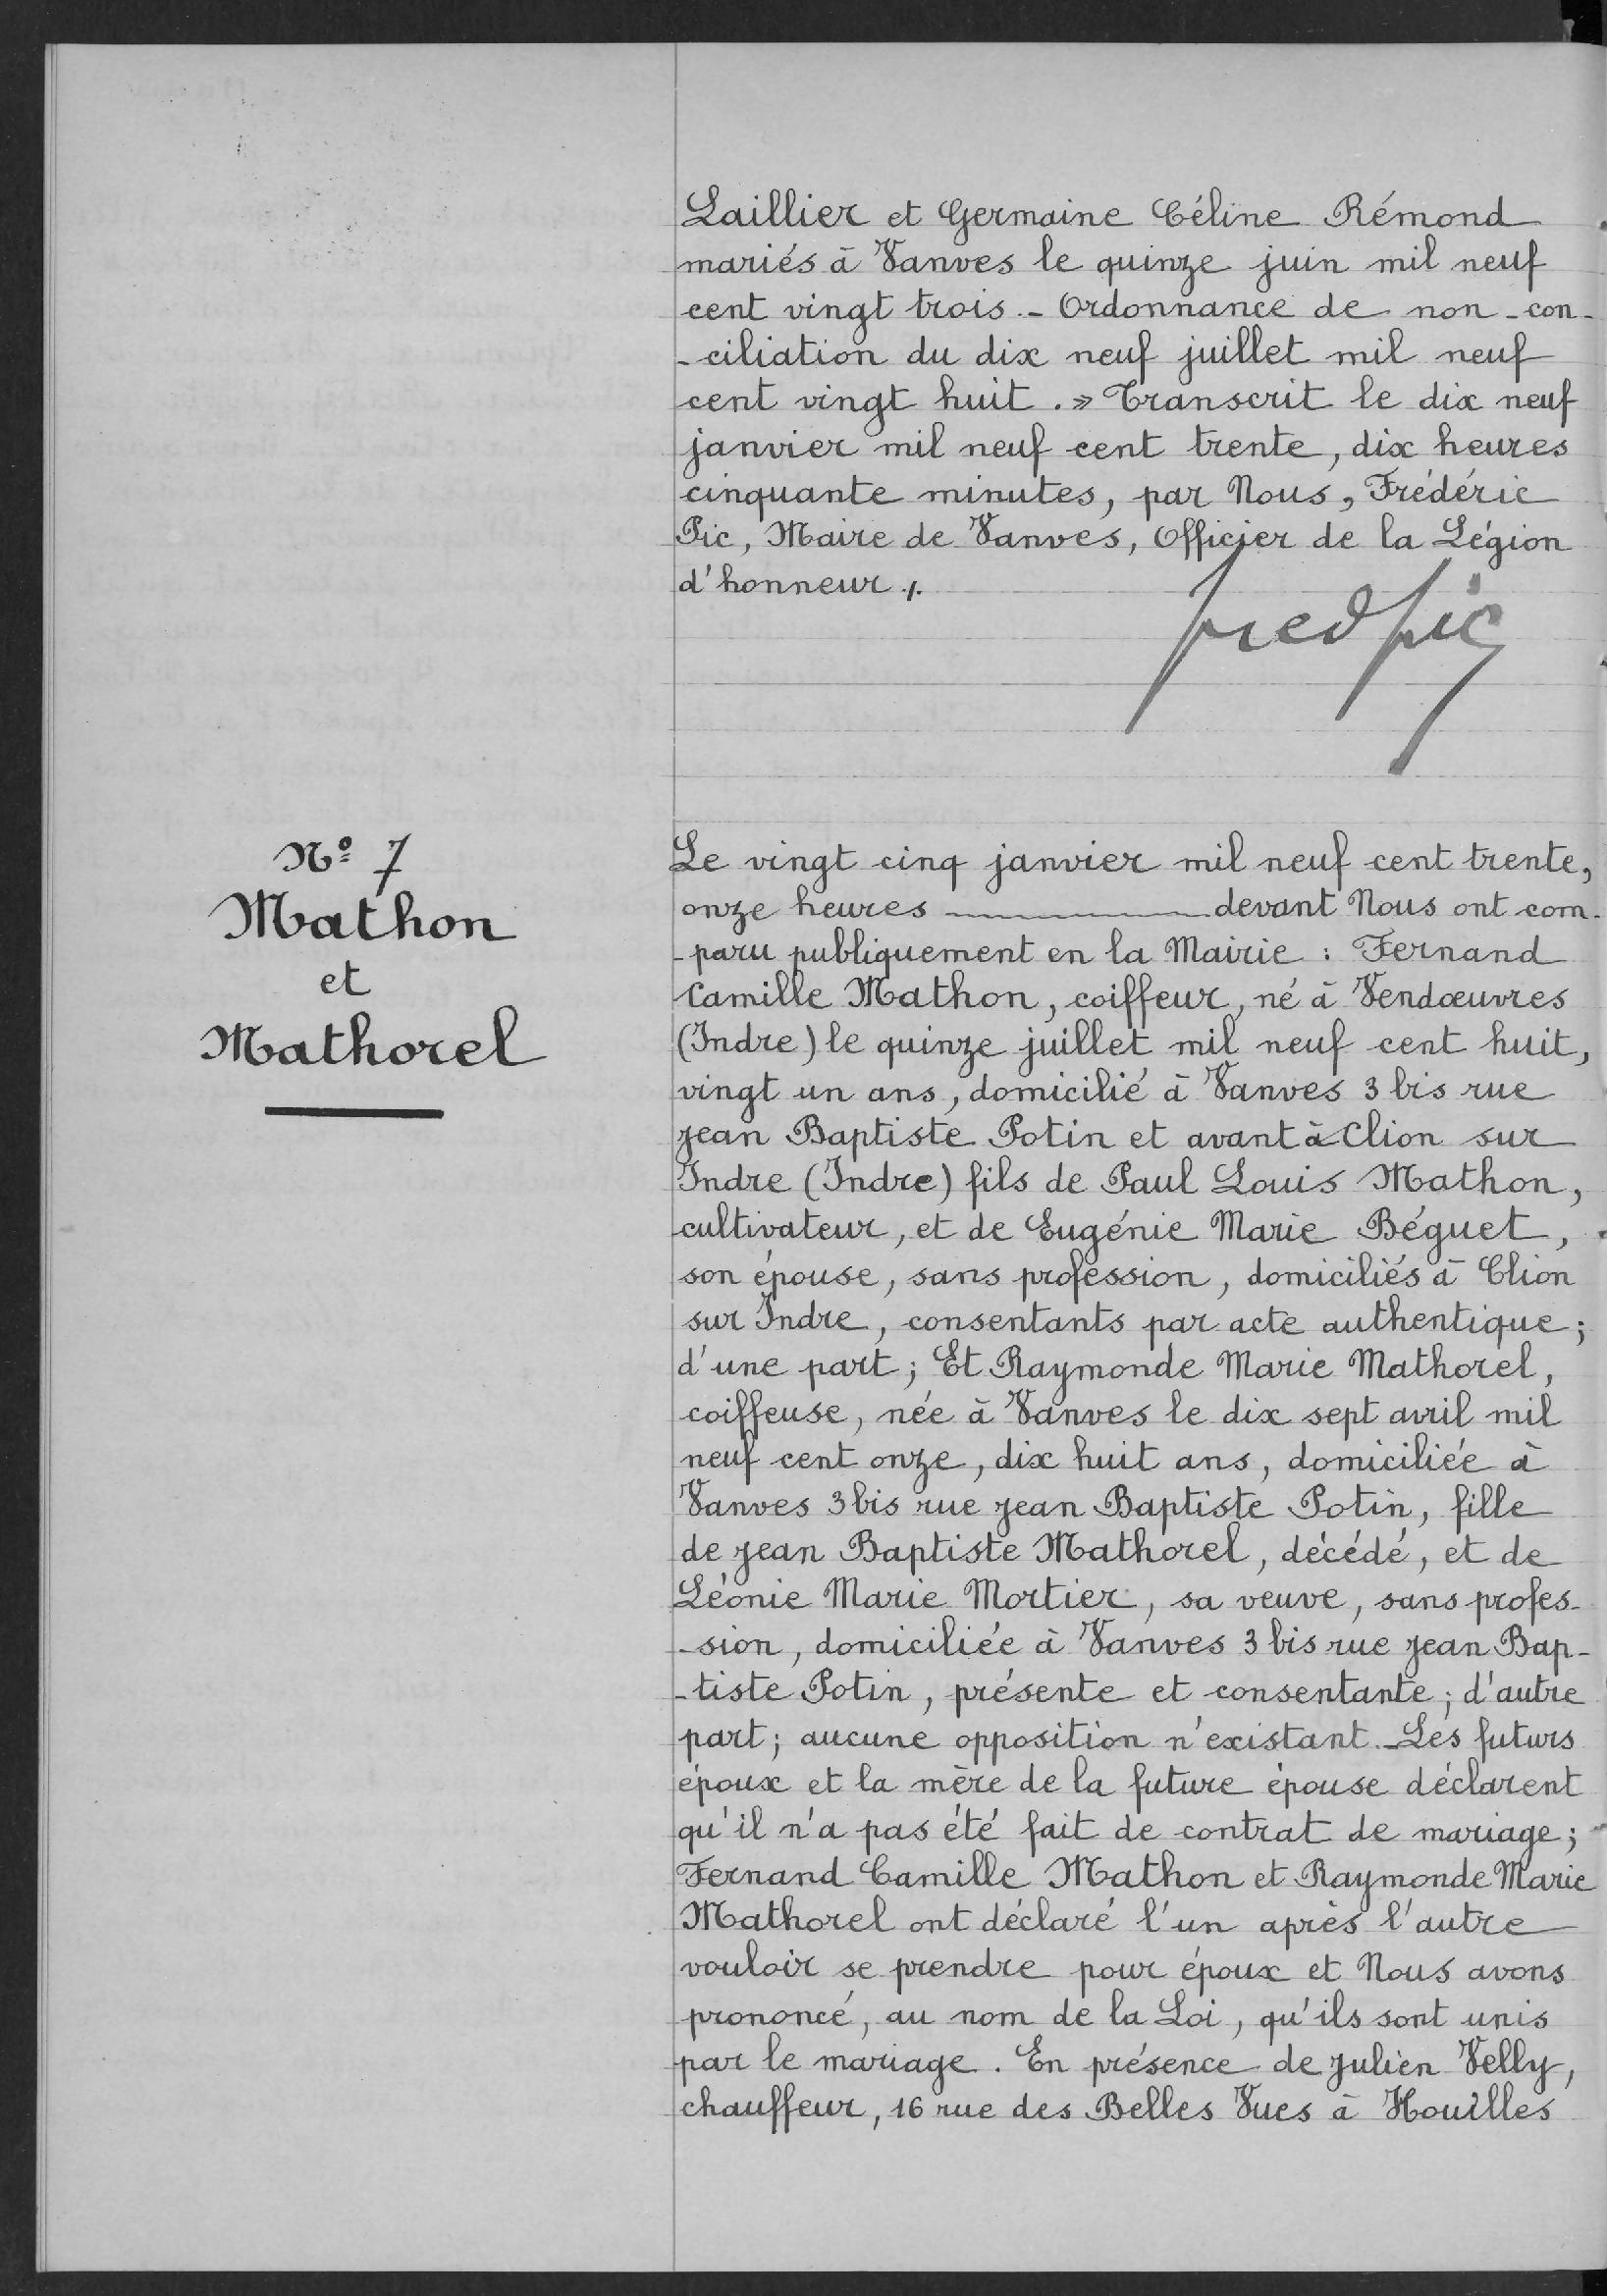Laillier et Germaine Céline Rémond
mariés à Vanves le quinze juin mil neuf
cent vingt trois.-Ordonnance de non-con-
-ciliation du dix neuf juillet mil neuf
cent vingt huit." Transcrit le dix neuf
janvier mil neuf cent trente, dix heures
cinquante minutes, par Nous, Frédéric
Pic, Maire de Vanves, Officier de la Légion
d'honneur./.
N°7
Mathon
et
Mathorel
Le vingt cinq janvier mil neuf cent trente
onze heures -devant Nous ont com-
-paru publiquement en la Mairie: Fernand
Camille Mathon, coiffeur, né à Vendoeuvres
(Indre) le quinze juillet mil neuf cent huit
vingt un ans, domicilié à Vanves 3bis rue
Jean Baptiste Potin et avant à Clion sur
Indre (Indre) fils de Paul Loui Mathon,
cultivatuer, et de Eugénie Marie Béguet,
son épouse, sans profession, domiciliés à Clion
sur Indre, consentants par acte authentique;
d'une part; Et Raymonde Marie Mathorel,
coiffeuse; née à Vanves le dix sept avril mil
neuf cent onze, dix huit ans, domiciliée à
Vanves 3 bis rue Jean Baptiste Potin, fille
de Jean Baptiste Mathorel, décédé et de
Léonie Marie Mortier, sa veuve, sans profes-
-sion, domiciliée à Vanves 3 bis rue Jean Bap-
-tiste Potin, présente et consentante; d'autre
part; aucune opposition n'existant. -Les futurs
époux et la mère de la future épouse déclarent
qu'il n'a pas été fait de contrat de mariage;
Fernand Camille Mathon et Raymonde Marie
Mathorel ont déclaré l'un après l'autre
vouloir de prendre pour époux et Nous avons
prononcé, au nom de la Loi, qu'ils sont unis
par le mariage. En présence de Julien Velly,
chauffeur, 16 rue des Belles Vues à Houilles
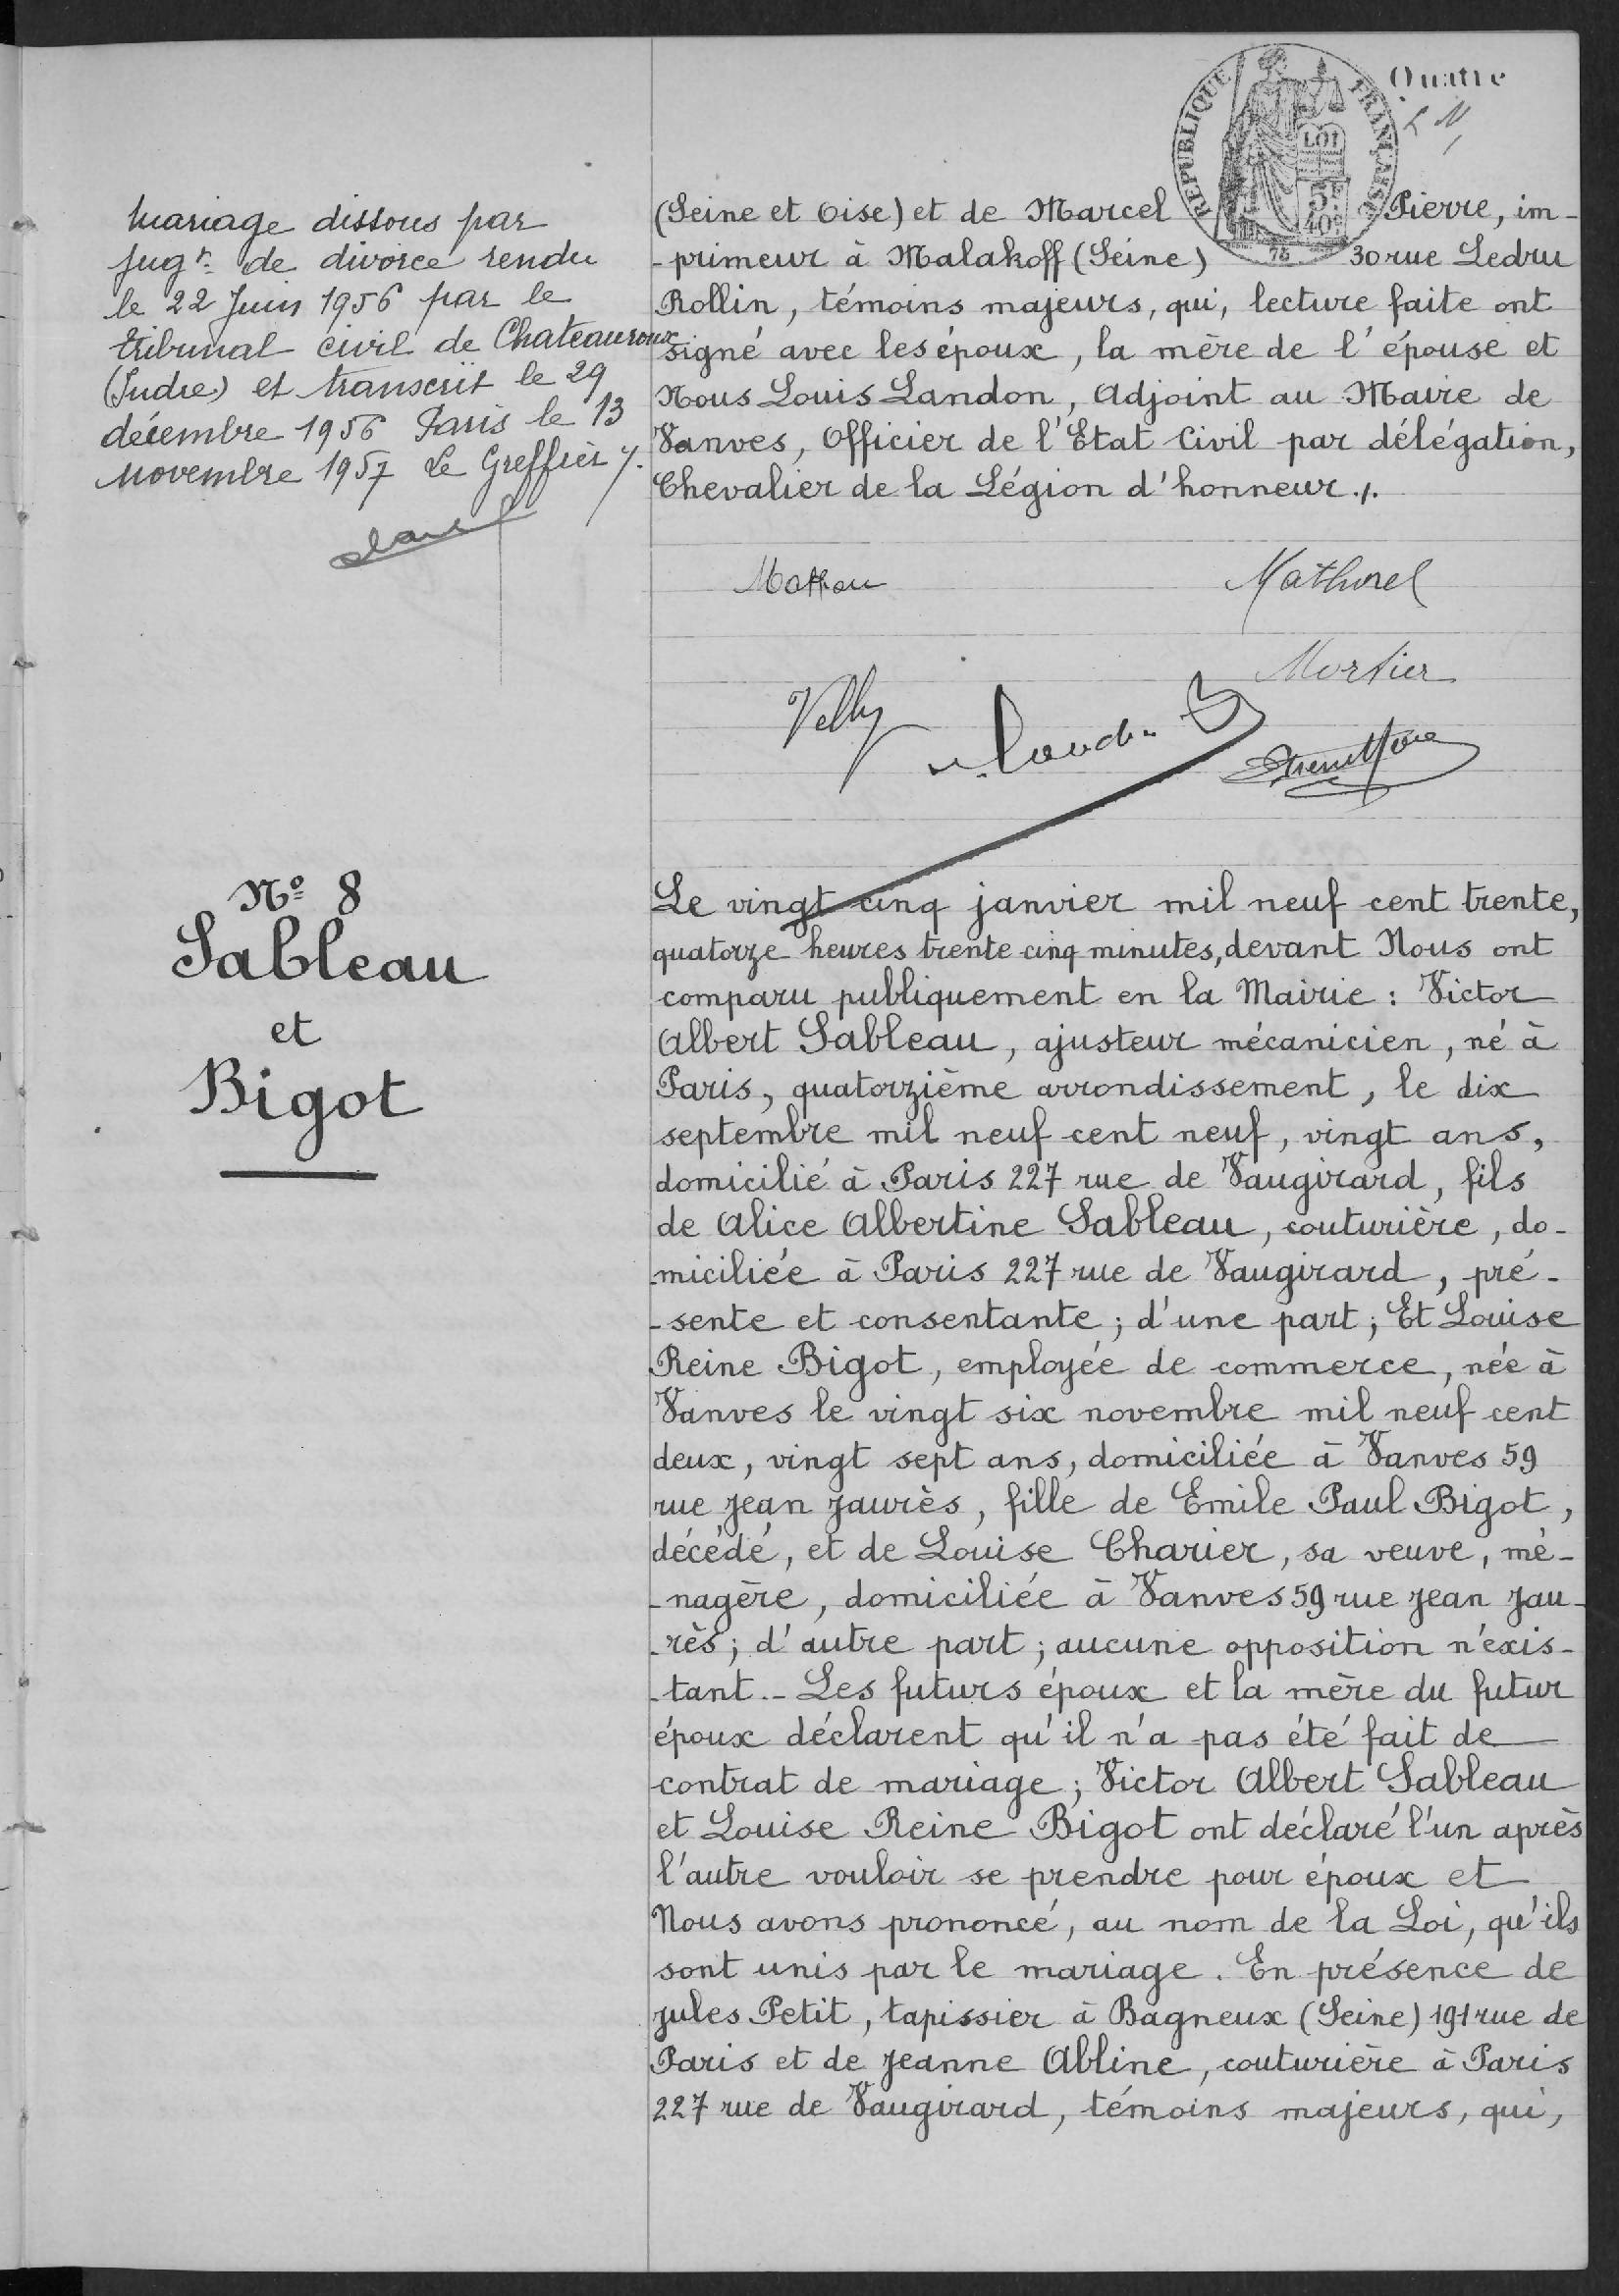(Seine et Oise) et de Marcel Pierre, im
-primeur à Malakoff (Seine) 30 rue Ledru
Rollin, témoins majeurs, qui, lecture faite ont
signé avec les époux, la mère de l'épouse et
nous Louis Landon, Adjoint au Maire de
Vanves, Officier de l'Etat Civil par délégation,
Chevalier de la Légion d'Honneur./.
n°8
Sableau
et
Bigot
Le vingt cinq janvier mil neuf cent trente,
quatorze heures trente cinq minutes, devant Nous ont
comparu publiquement en la Maire: Victore
Albert Sableau, ajusteur mécanicien, né à
Paris, quatorzième arrondissement, le dix
septembre mil neuf-cent neuf, vingt ans,
domicilié à Paris 227 rue de Vaugirard, fils
de Alix Albertine Sableau, couturière, do-
miciliée à Paris 227 rue de Vaugirard, pré-
-sente et consentante; d'une part; et Louise
Reine Bigot, employée de commerce, née à
Vanves le vingt six novembre mil neuf cent
deux, vingt sept ans, domiciliée à Vanves 59
rue, Jean Jaurès, fille de Emile Paul Bigot,
décédé, et de Louise Charier, sa veuve, mé-
-nagère, domiciliée à Vanves, 59 rue Jean Jau-
-rès; d'autre part; aucune opposition n'exis-
-tant.-Les futurs époux et la mère du futur
époux déclarent qu'il n'a pas été fait de -
contrat de mariage; Victor Albert Sableau
et Louise Reine Bigot ont déclaré l'un après
l'autre vouloir se prendre pour époux et
Nous avons prononcé, au nom de la Loi, qu'ils
sont unis par le mariage. En présence de
Jules Petit, tapissier à Bagneux (Seine) 191 rue de
Paris et de Jeanne Abline, couturière à Paris
227 rue de Vaugirard, témoins majeurs, qui,